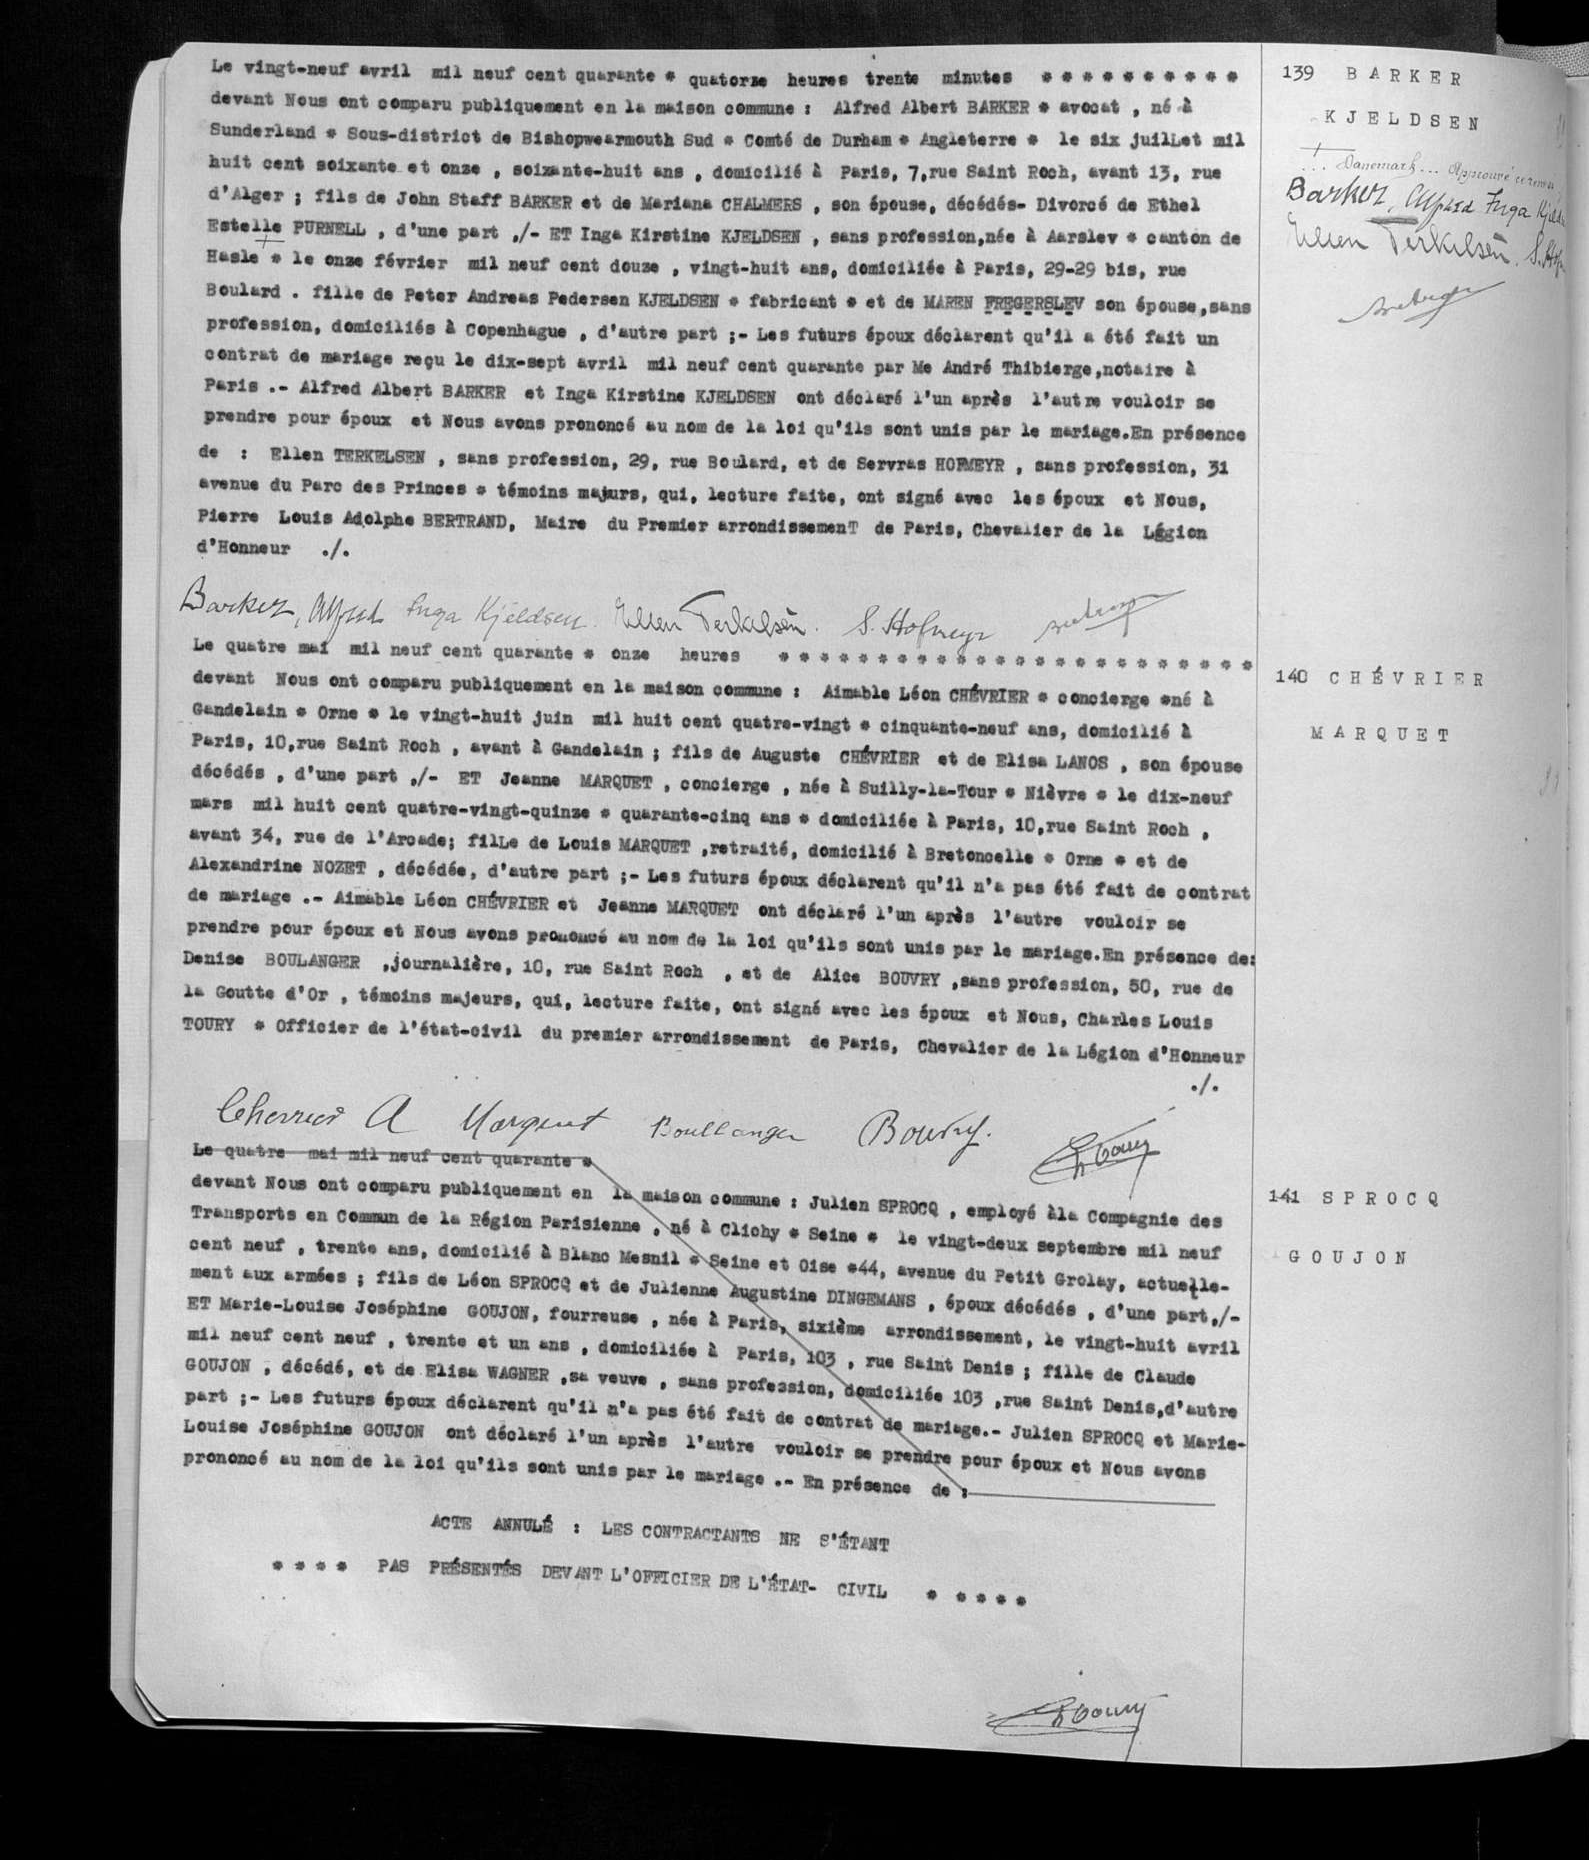Le vingt-neuf avril mil neuf cent quarante * quatorze heure trente minutes ****
devant Nous ont comparu publiquement en la maison commune: Alfred Albert BARKER * avocat, né à
Sunderland * Sous-district de Bishpwearmouth Sud * Comté de Durham * Angleterre * le six juillet mil
huit cent soixante et onze, soixante-huit ans, domicilié à Paris, 7, rue Saint Roch, avant 13, rue
d'Alger; fils de John Staff BARKER et de Mariana CHALMERS, son épouse, décédés-Divorcé de Ethel
Estelle PURNELL, d'une part ,/-ET Inga Kirstine KJELDSEN, sans profession, née à Aarslev * canton de
Hasle * le onze février mil neuf cent douze, vingt-huit ans, domiciliée à Paris, 29-29 bis, rue
Boulard. fille de Peter Andreas Pedersen KJELDSEN * fabricant * et de MAREN FREGERSLEV son épouse, sans
profession, domiciliés à Copenhague, d'autre part ;-Les futurs époux déclarent qu'il a été fait un
contrat de mariage reçu le dix-sept avril mil neuf cent quarante par Me André Thibierge, notaire à
Paris .-Alfred Albert BARKER et Inga Kirstine KJELDSEN ont déclaré l'un après l'autre vouloir se
prendre pour époux et Nous avons prononcé au nom de la loi qu'ils sont unis par le mariage. En présence
de: Ellen TERKELSEN, sans profession, 29, rue Boulard, et de Servras HOFMEYR, sans profession, 31
avenue du Parc des Princes * témoins majeurs, qui, lecture faite, ont signé avec les époux et Nous,
Pierre Louis Adolphe BERTRAND, Maire du Premier arrondissemenT de Paris, Chevalier de la Lggion
d'Honneur ./.
Le quatre mai mil neuf cent quarante * onze heures ****
devant Nous ont comparu publiquement en la maison commune: Aimable Léon CHEVRIER * concierge * né à
Gandelain * Orne * le vingt-huit juin mil huit cent quatre-vingt * cinquante-neuf ans, domicilié à
Paris, 10, rue Saint Roch, avant à Gandelain; fils de Auguste CHEVRIER et de Elisa LANOS, son épouse
décédés, d'une part ,/-ET Jeanne MARQUET, concierge, née à Suilly-la-Tour * Nièvre * le dix-neuf
mars mil huit cent quatre-vingt-quinze * quarante-cinq ans * domiciliée à Paris, 10, rue Saint Roch,
avant 34, rue de l'Arcade; filLe de Louis MARQUET, retraité, domicilié à Bretoncelle * Orne * et de
Alexandrine NOZET, décédée, d'autre part ;-Les futurs époux déclarent qu'il n'a pas été fait de contrat
de mariage .-Aimable Léon CHEVRIER et Jeanne MARQUET ont déclaré l'un après l'autre vouloir se
prendre pour époux et Nous avons prononcé au nom de la loi qu'ils sont unis par le mariage. En présence
des Denise BOULANGER, journalière, 10, rue Saint Roch, et de Alice BOUVRY, sans profession, 50, rue de
la Goutte d'Or, témoins majeurs, qui, lecture faite, ont signé avec les époux et Nous, Charles Louis
TOURY * Officier de l'état-civil du premier arrondissement de Paris, Chevalier de la Légion d'Honneur ./.
Le quatre mai mil neuf cent quarante *
devant Nous ont comparu publiquement en la maison commune: Julien SPROCQ, employé à la Compagnie des
Transports en Commun de la Région Parisienne, né à Clichy * Seine * le vingt-deux septembre mil neuf
cent neuf, trente ans, domicilié à Blanc Mesnil * Seine et Oise * 44, avenue du Petit Grolay, actuelle-
ment aux armées; fils de Léon SROCQ et de Julienne Augustine DINGEMANS, époux décédés, d'une part ,/
ET Marie-Louise Joséphine GOUJON, fourreuse, née à Paris, sixième arrondissement, le vingt-huit avril
mil neuf cent neuf, trente et un ans, domiciliée à Paris, 103, rue Saint Denis; fille de Claude
GOUJON, décédé, et de Elisa WAGNER, sa veuve, sans profession, domiciliée 103, rue Saint Denis, d'autre
part ;-Les futurs époux déclarent qu'il n'a pas été fait de contrat de mariage .-Julien SPROCQ et Marie-
Louis Joséphine GOUJON ont déclaré l'un après l'autre vouloir se prendre pour époux et Nous avons
prononcé au nom de la loi qu'ils sont unis par le mariage .-En présence de:
ACTE ANNULE: LES CONTRACTANTS NE S'ETANT
**** PAS PRESENTES DEVANT L'OFFICIER DE L'ETAT-CIVIL ****
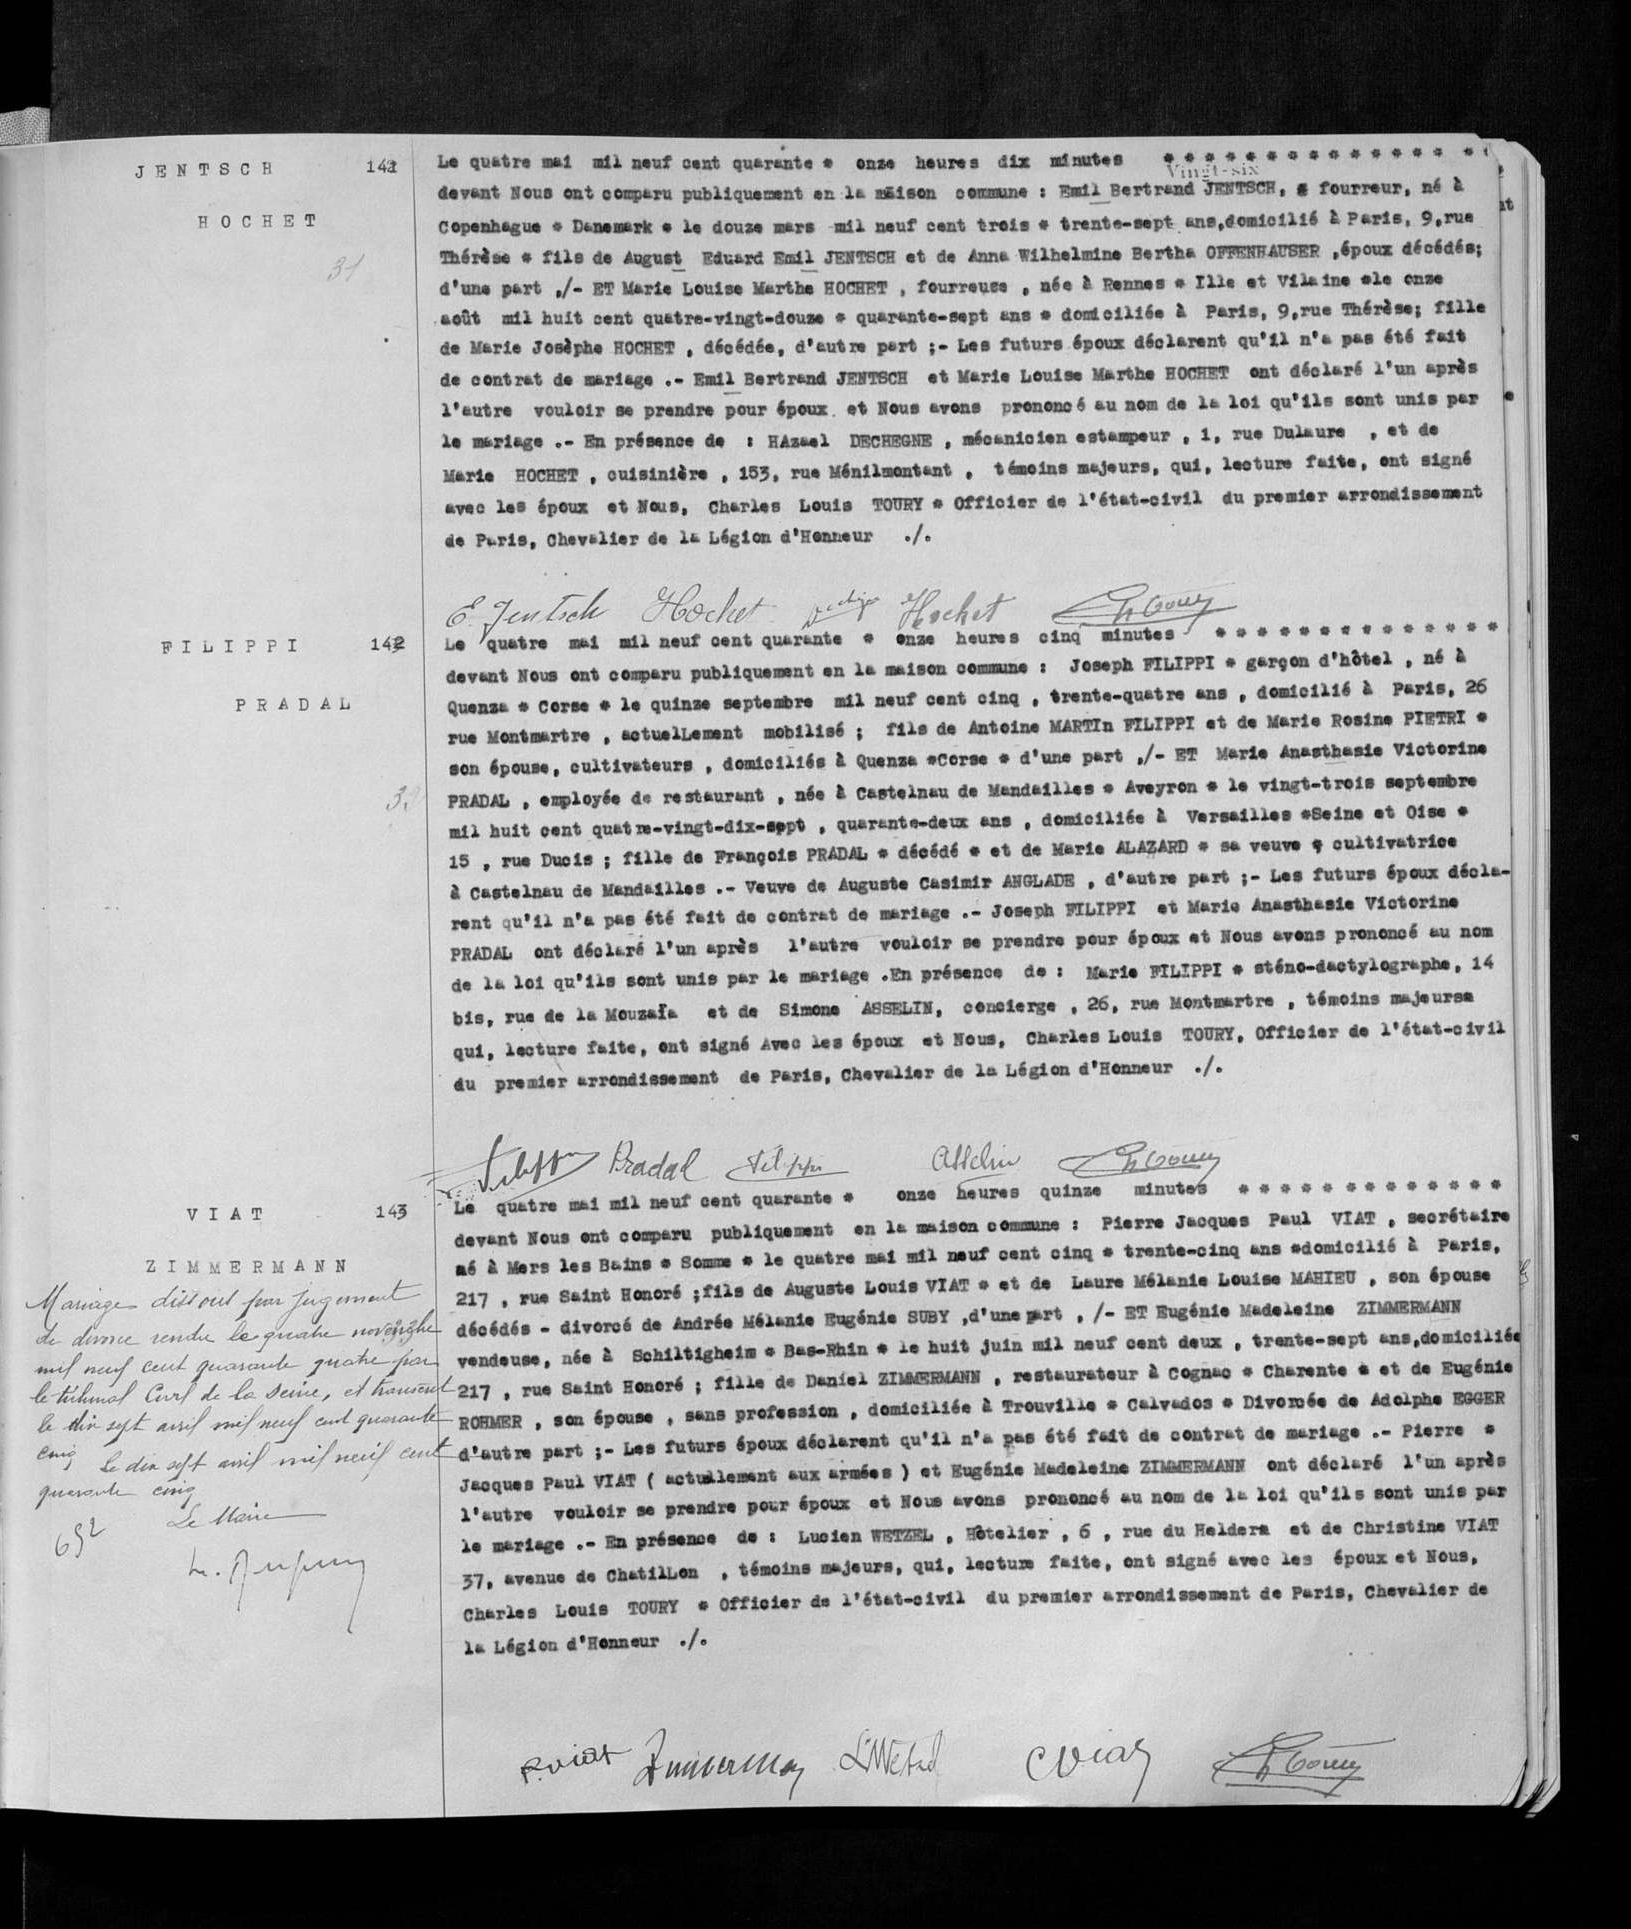Le quatre mai mil neuf cent quarante * onze heures dix minutes ****
devant Nous ont comparu publiquement en la maison commune: Emil Bertrand JENTSCH, fourreur, né à
Copenhague * Danemark * le douze mars mil neuf cent trois * trente-sept ans, domicilié à Paris, 9, rue
Thérèse * fils de August Eduard Emil JENTSCH et de Anna Wilhelmine Bertha OFFENHAUSER, époux décédés;
d'une part ,/-ET Marie Louise Marthe HOCHET, fourreuse, née à Rennes * Ille et Vilaine * le onze
août mil huit cent quatre-vingt-douze * quarante-set ans * domiciliée à Paris, 9, rue Thérèse; fille
de Marie Josèphe HOCHET, décédée, d'autre part ;-Les futurs époux déclarent qu'il n'a pas été fait
de contrat de mariage .-Emil Bertrand JENTSCH et Marie Louise Marthe HOCHET ont déclaré l'un après
l'autre vouloir se prendre pour époux et Nous avons prononcé au nom de la loi qu'ils sont unis par
le mariage .-En présence de: HAzael DECHEGNE, mécanicien estampeur, 1, rue Dulaure, et de
Marie HOCHET, cuisinière, 153, rue Ménilmontant, témoins majeurs, qui, lecture faite, ont signé
avec les époux et Nous, Charles Louis TOURY * Officier de l'état-civil du premier arrondissement
de Paris, Chevalier de la Légion d'Honneur ./.
Le quatre mai mil neuf cent quarante * onze heures cinq minutes ****
devant Nous ont comparu publiquement en la maison commune: Joseph FILIPPI * garçons d'hôtel, né à
Quenza * Corse * le quinze septembre mil neuf cent cinq, trente-quatre ans, domicilié à Paris, 26
rue Montmartre, actuellement mobilisé; fils de Antoine MARTIn FILIPPI et de Marie Rosine PIETRI *
son épouse, cultivateurs, domiciliés à Quenza * Corse * d'une part ,/-ET Marie Ansthasie Victorine
PRADAL, employée de restaurant, née à Castelnau de Mendailles * Aveyron * le vingt-trois septembre
mil huit cent quatre-vingt-dix-sept, quarante-deux ans, domiciliée à Versailles * Seine et Oise *
15, rue Ducis; fille de François PRADAL * décédé * et de Marie AlAZARD * sa veuve * cultivatrice
à Castelnau de Mendailles .-Veuve de Auguste Casimir ANGLADE, d'autre part ;-Les futurs époux décla-
rent qu'il n'a pas été fait de contrat de mariage .-Joseph FILIPPI et Marie Anasthasia Victorine
PRADAL ont déclaré l'un après l'autre vouloir se prendre pour époux et Nous avons prononcé au nom
de la loi qu'ils sont unis par le mariage. En présence de: Marie FILIPPI * sténo-dactylographe, 14
bis, rue de la Mouzafa et de Simone ASSELIN, concierge, 26, rue Montmartre, témoins majeurs
qui, lecture faite, ont signé Avec les époux et Nous, Charles Louis TOURY, Officier de l'état-civil
du premier arrondissement de Paris, Chevalier de la Légion d'Honneur ./.
Le quatre mai mil neuf cent quarante * onze heures quinze minutes ****
devant Nous ont comparu publiquement en la maison commune: Pierre Jacque Paul VIAT, secrétaire
né à Mers les Bains * Somme * le quatre mai mil neuf cent cinq * trente-cinq ans * domicilié à Paris,
217, rue Saint Honoré; fils de Auguste Louis VIAT * et de Laure Mélanie Louise MAHIEU, son épouse
décédés-divorcé de Andrée Mélanie Eugénie SUBY, d'une part, /-ET Eugénie Madeleine ZIMMERMANN
vendeuse, née à Schiltigheim * Bas-Rhin * le huit juin mil neuf cent deux, trente-sept ans, domiciliée
217, rue Saint Honoré; fille de Daniel ZIMMERMANN, restaurateur à Cognac * Charente * et de Eugénie
ROHMER, son épouse, sans profession, domiciliée à Trouville * Calvados * Divorcée de Adolphe EGGER
d'autre part ;-Les futurs époux déclarent qu'il n'a pas été fait de contrat de mariage .-Pierre *
Jacques Paul VIAT (actuellement aux armées) et Eugénie Madeleine ZIMMERMANN ont déclaré l'un après
l'autre vouloir se prendre pour époux et Nous avons prononcé au nom de la loi qu'ils ont unis par
le mariage .-En présence de: Lucien WETZEL, Hôtelier, 6, rue du Helder et de Christin VIAT
37, avenue du ChatilLon, témoins majeurs, qui, lecture faite, ont signé avec les époux et Nous,
Charles Louis TOURY * Officier de l'état-civil du premier arrondissement de Paris, Chevalier de
la Légion d'Honneur ./.